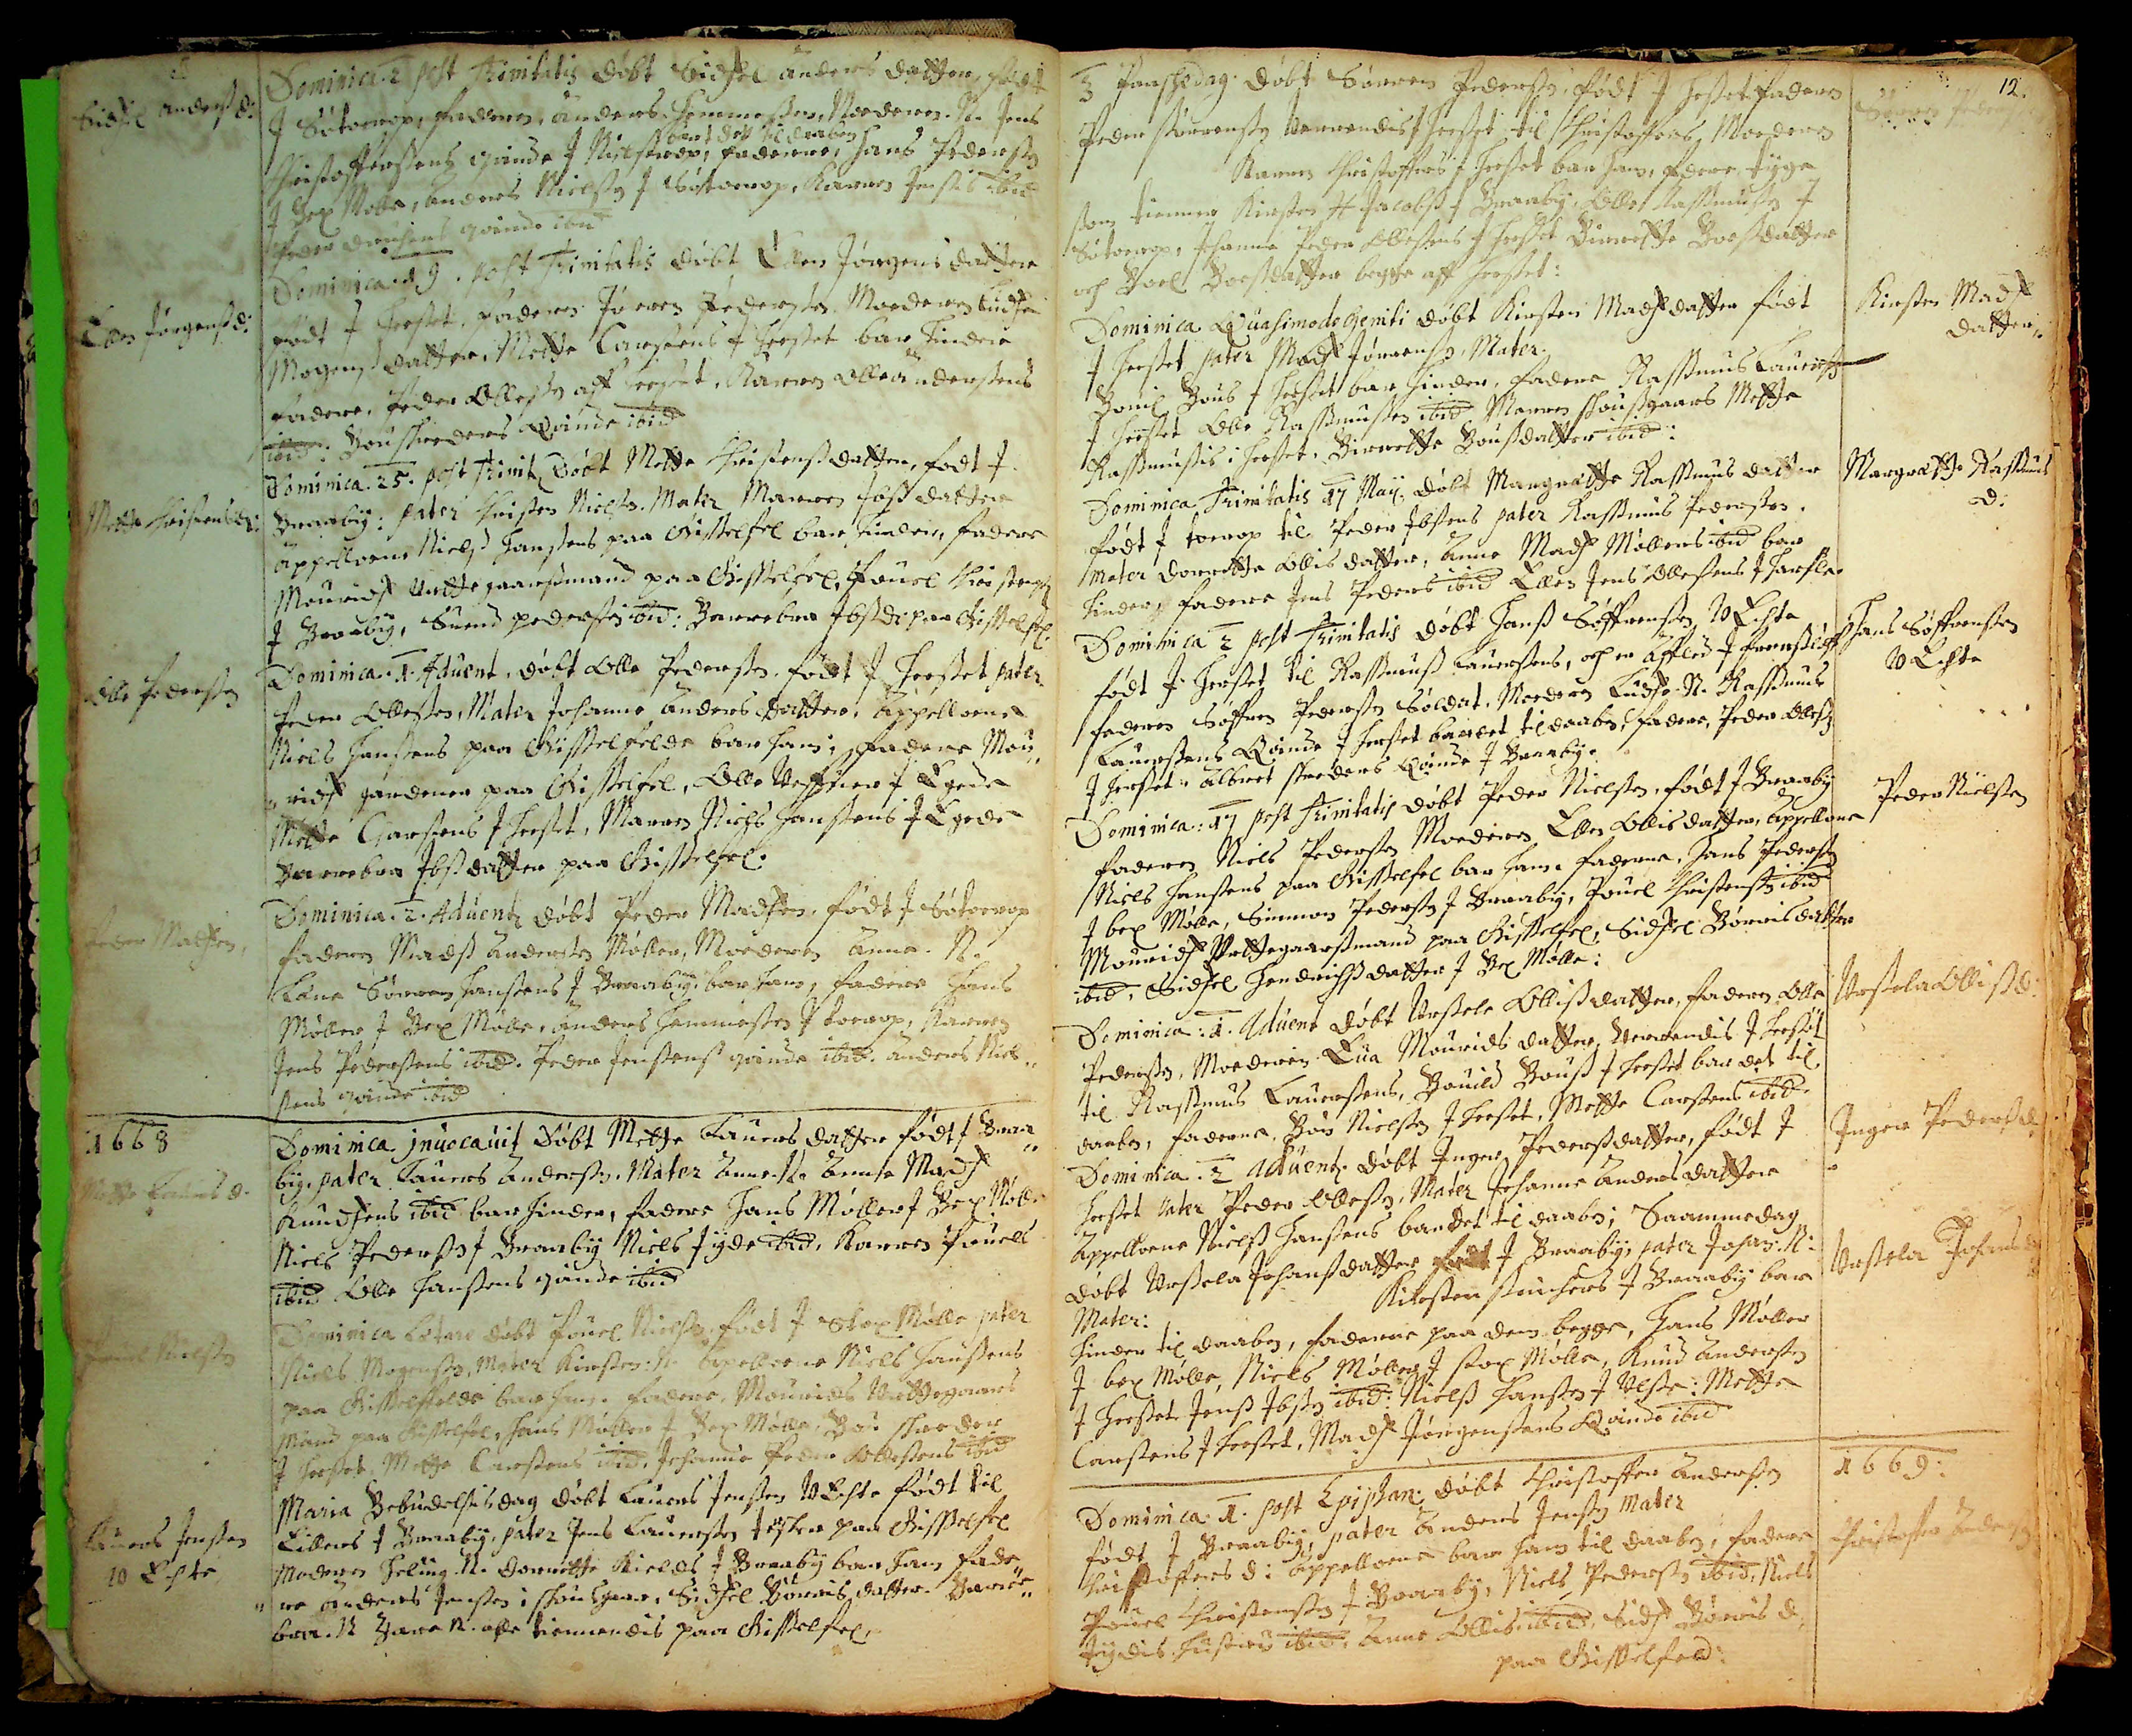Sidsel Andersd:
Dominica. 2 post Trinitatis døbt Sidsel Anders datter født
J Søtoerup. Faderen Anders Hemmesen. Modern N. Jens.
bar det til daaben
Christoffersens Qvinde J Nielstrup Faderne, Hans Pedersen
J Bex Mølle, Anders Nielsen J Søtorup, Karren Jensd. ibid.
Peder Drusens Qvinde ibid.
Ellen Jørgensd:
Dominica d. 9 post Trinitatis døbt Ellen Jørgens datter 
født J Herset. Faderen Jørren Pedersen. Moderen Ludse 
Mogensdatter Mette, Carstens J Heeset bar hinder. 
Fadere, Peder Ollesen aff Herset, Karren Olle Andersens 
ibid. Bou Skreders Qvinde ibid.
Mette Christensd:
Dominica. 25. post Trinit Døbt Mette Christensdatter født J
Braaby. Pater Christen Nielsen. Mater Marren Josdatter
Appellone Niels Hansens paa Gisselfel bar hinder. Fadrere
Mauridz Urttegaardsmand paa Gisselfel, Pouel Christensen
J Braaby, Suend Pedersen ibid. Barrebra Jbsd. paa Gisselfel.
Olle Pedersen 
Dominica. 1. Aduent døbt Olle Pedersen født J Heeset. Pater 
Peder Ollesen, Mater Johanne Anders datter. Appellone 
Niels Hansens paa Gisselfelde bar ham: Fadere, Mou¬
rids gardener paa Gisselfel, Olle Veffer J Egede
Mette Carstens J Heset. Marren Niels Hansens J Egede
Barrebra Jbsdatter paa Gisselfel:
Peder Madzen
Dominica 2. Aduentz døbt Peder Madzen født J Søtorup
Faderen Mads Andersen Møller, moderen Anna N. 
Læne Sørren Hansens J Braaby bar ham, Fadere Hans
Møller J Bex Mølle, Anders Hemmisen J Torup, Karren 
Jens Pedersens ibid. Peder Jensens qvinde ibid. Anders Niel
sens qvinde ibid.
1668 
Mette Lauersd. 
Dominica. jnvocavit døbt Mette Lauers datter født J Braa¬
by. Pater Lauers Andersen. Mater Anna N., Anna Madz 
Knudzens ibid. bar hinder, Fadere Hans Møller J Bexmølle,
Niels Pedersen J Braaby, Niels Jyde ibid. Karren Pouels
ibid. Olle Hansens qvinde ibid.
Pouel Nielsen 
Dominica Lætare døbt Pouel Nielsen født J Stox Mølle Pater 
Niels Mogensen, Mater Kirsten N. Appellone Niels Hansens 
paa Gisselfelde bar ham. Fadere, Mourids Urttegaards 
mand paa Gisselfel, Hans Møller J Bex Mølle, Bou shreder 
J Herset, Mette Carstens ibid. Johanne Peder Ollesens ibid.
Lauers Jensøn 
U Echte 
Maria Bebudelsisdag døbt Lauers Jensen U Echte. født til 
Eillers J Braaby, Pater Jens Lauersen fisker paa Gisselfel
Modern Heliug N. Dorrette Kields J Braaby bar ham, Fader¬
ne Anders Jensen i Skousgaar, Sidsel Børrisdatter. Barre¬
bra N. Lhene N## alle tiennendis paa Gisselfel.
3 Paaskedag døbt Sørren Pedersen, født i Hesset, Faderen 
Peder Sørrensen verrendis J Herset til Christoffers, Moderen
Karren Christoffers i Heeset bar ham, Fadere Tyge
som tienner Kirsten H. Jacobs J Braaby, Olle Rassmussen J
Søtoerop, Johanne Peder Ollesens J Heeset, Birrette Boesdatter 
og Boel Boesdatter begge aff Heeset: 
12.
Sørren Pedersen
Dominica Quasimodo Geniti døbt Kirsten Madzdatter født 
J Heeset. Pater Mads Jørrensen, Mater.
Bodil Bous J Heeset bar hinder, Fadere Rassmus Laurits
J Heeset, Olle Rassmusen ibid. Marren Skousgaars, Mette
Rassmusis i Heeset, Birrette Bousdatter ibid:
Kirsten Madz 
datter 
Dominica Trinitatis 17 May døbt Margrette Rassmus datter
født J Toerop til Peder Jbsens, Pater Rassmus Pedersen. 
Mater Dorrette Ollis datter, Anne Mads Møllers ibid. bar
hinder, Fadere Jens Peders ibid. Ellen Jens Ollesens J Harsle.
Margrette Rassmus 
d:
Dominica 2 post Trinitatis døbt Hans Søffrensen U Echte 
født J Herset til Rassmus Lauersens, och er Affled## J Freerslef##.
Faderen Søffren Pedersen Søldat. Moedern Ludze N. Rassmus
Lauersens Qvinde J Herset bar det til daaben, Fadere, Peder Ollesen 
J Herset, Albret shreders Qvinde J Braaby.
Hans Søffrensøn 
U Echte
Dominica: 17 post Trinitatis døbt Peder Nielsen, født J Braaby,
Faderen Niels Pedersen, Moederen Ellen Ollisdatter, Appelone 
Niels Hansens paa Gisselfel bar ham, Faderne, Jens Pedersen 
J Bex Mølle, Sørren## Pedersen J Braaby, Pouel Christensen ibid.
Maurids Urttegaarsmand paa Gisselfel, Sidzel Børrisdatter 
ibid. Sidzil Hendrichs datter J Bex Mølle:
Peder Nielsen 
Dominica: 1. Aduent døbt Ursela Ollisdatter, Faderen Olle
Pedersøn, Moederen Eua Mourids datter, Verrendis J heeset
til Rassmus Lauersens, Bouild Bous J heeset bar det til
daaben, Faderne Bou Nielsen i heeset, Mette Carstens ibid.
Ursela Ollisd:
Dominica. 2 Aduent døbt Jnger Pedersdatter, født i 
Heeset, Pater Ollesen, Mater Johanne Andersdatter 
Appelloene Niels Hansens bar det til daaben;
Jnger Pedersd
Samme dag
døbt Ursela Johansdatter født J Braaby; Pater Johan N:
Mater:
Kirsten sauchers## J Braaby bar
hinder til daaben, Faderer paa dem begge, Hans Møller
J Bex Mølle, Niels Møller J Stox Mølle, Knud Andersen
J Heeset, Jens Jbsen ibid: Niels Hansen J Ulse: Mette
Lausens J Heesed, Madz Jørgensens Qvinde ibid.
Ursela Johansd.
Dominica. 1. post Epiphan. døbt Christoffer Andersen
født J Braaby, Pater Anders Jensen. Mater   
Christoffersd: Appelloena bar ham til daaben, Fadere
Pouel Christensen J Braaby, Niels Pedersen ibid. Niels
Jydis Hustru ibid: Anne Ollis ibid. Sidsel## Børris d.
paa Gisselfeld:
1669:
Christoffer  Andersen